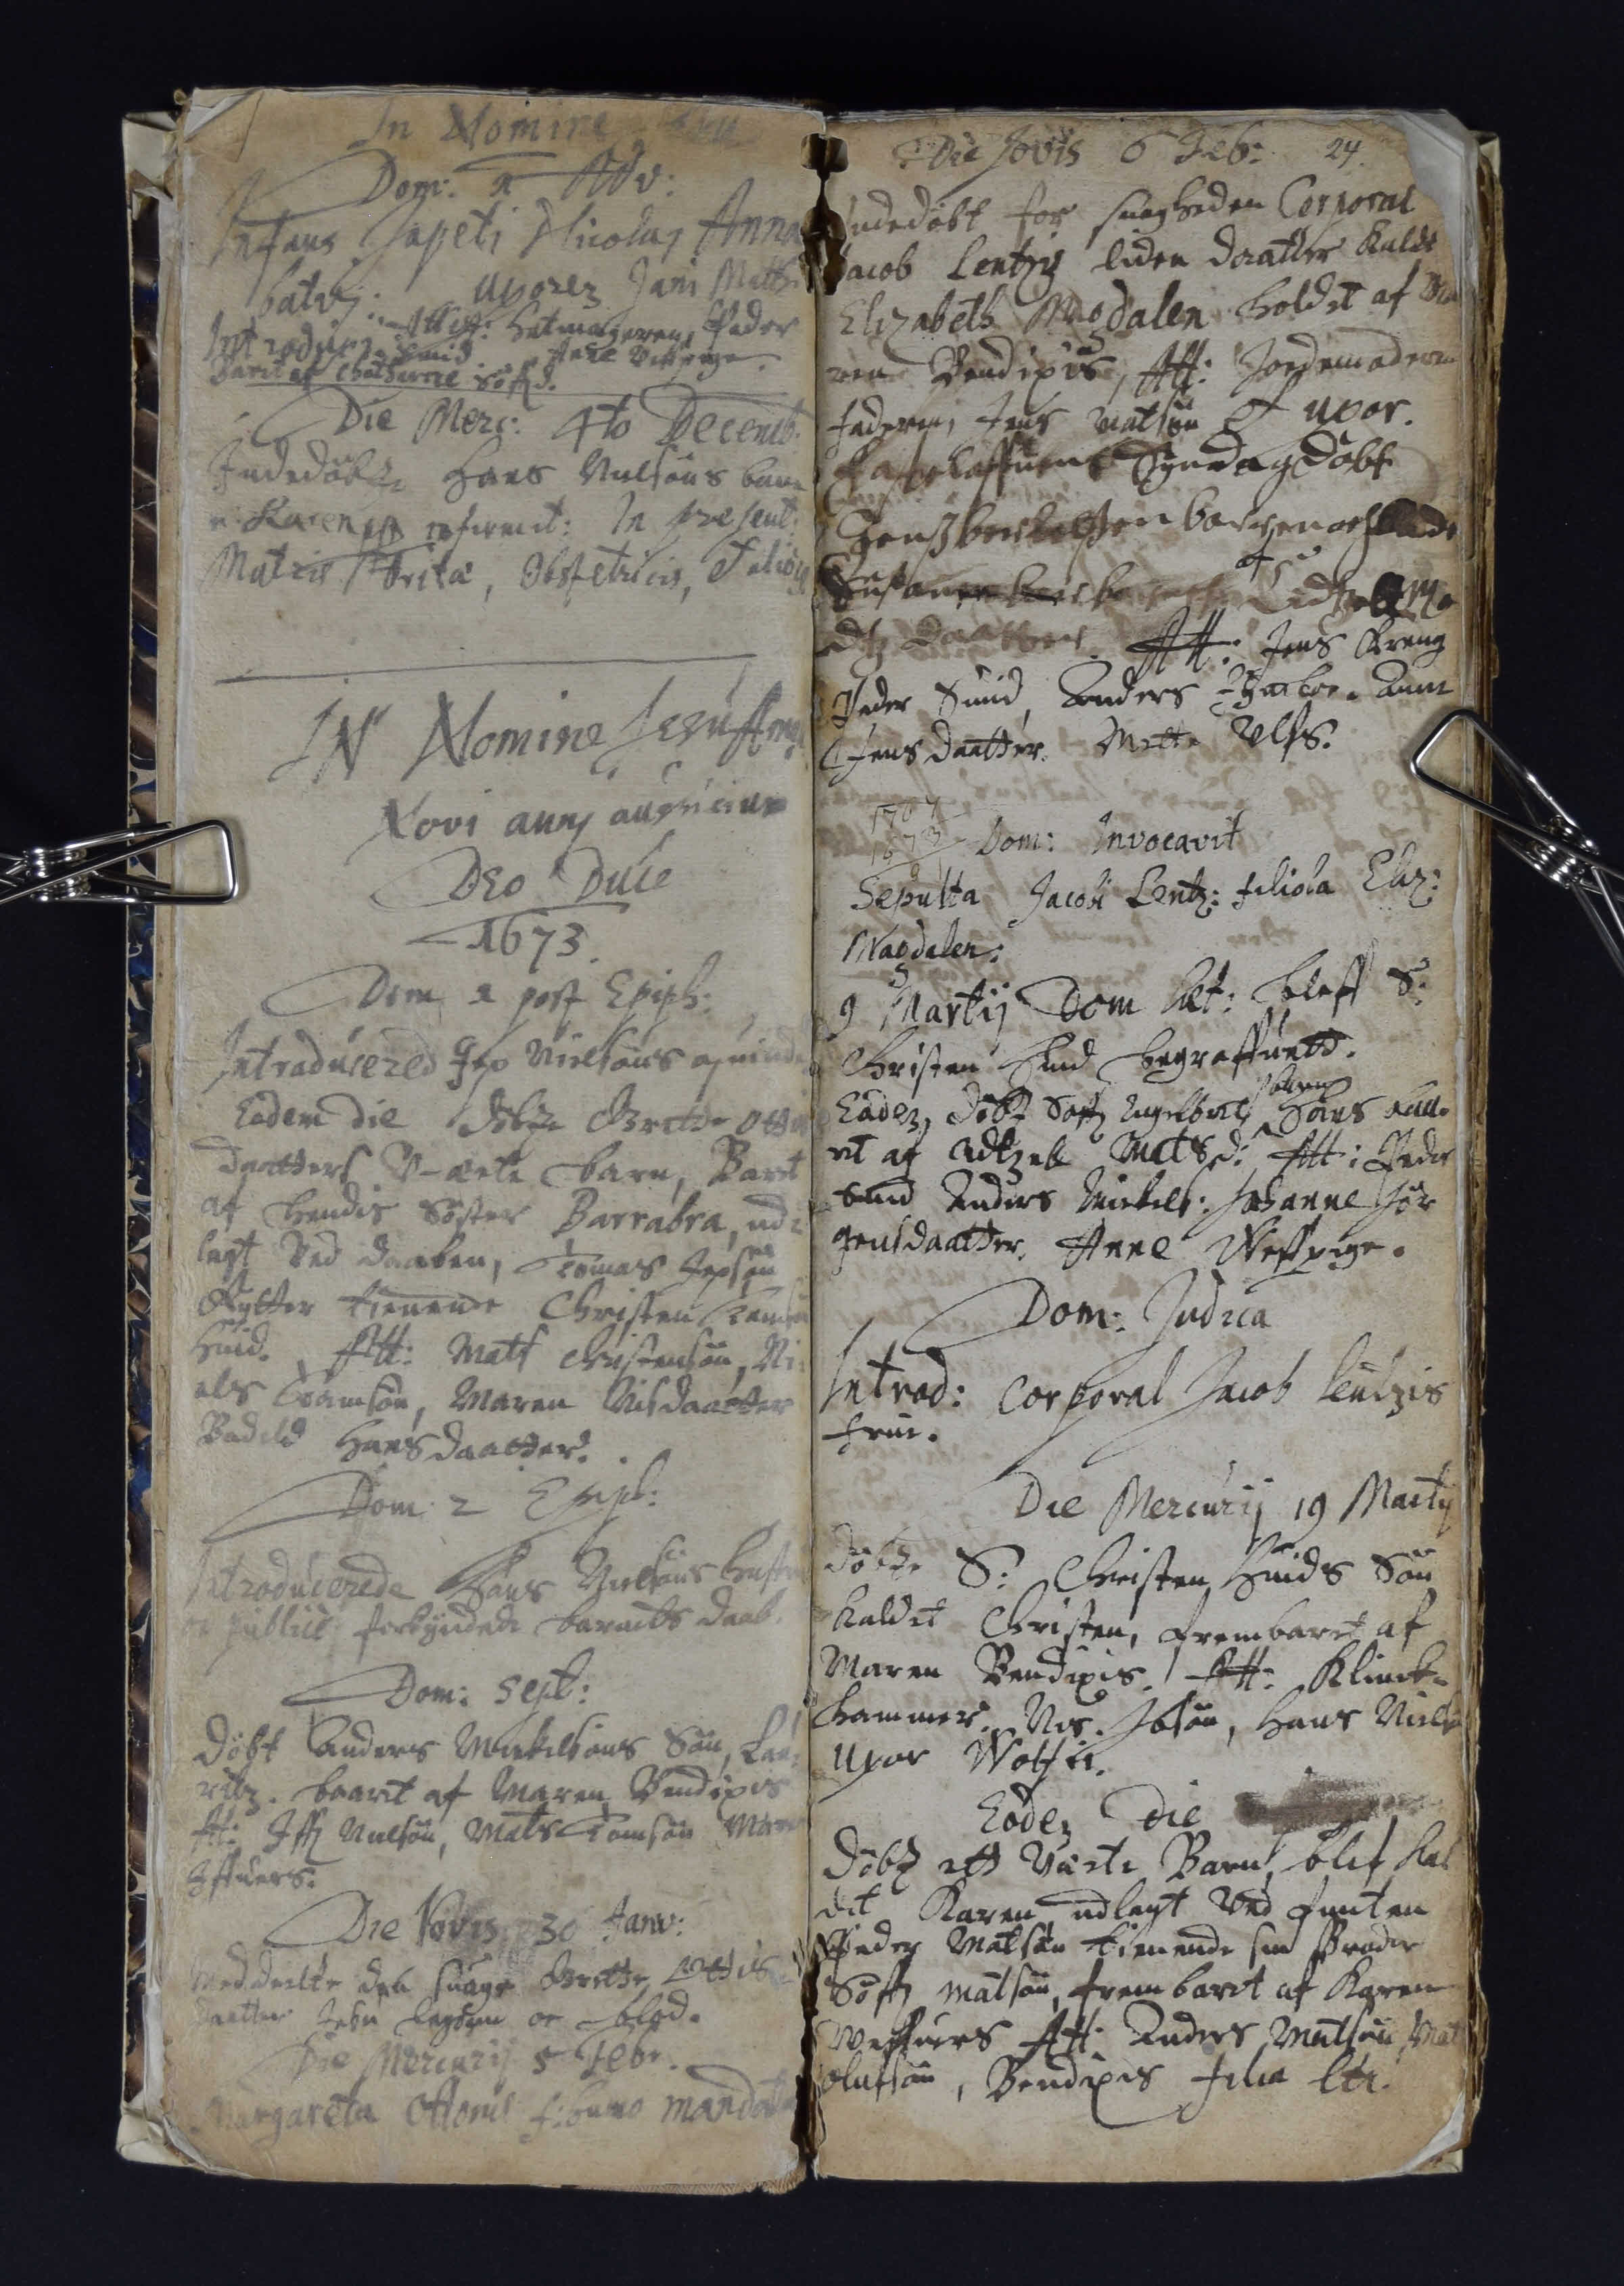In Nomine
Dom. 1 Adv: 
Infans Japeti Nicolaj Anna
uxorez Jani Matthiæ
batis i attest: Hatmaggeren. Peder
Introduci##.
Smid. Jens Ulfs pige
Karen oc Chatherine Søffd.
Die Merc: 4to Decemb
Indedøbte Hans Nielsøns barn
n: Karen, pp infirmit: In present:
Matris Hvita, Obstetricis,
IN Nomine  Jesu
Xovi anni
1673
DEO
Dom. 1 post Epiph:
Introducered Jep Nielsøns qvinde.
Eodem Die døbte Grette Ottis
daatters V-acte barn, Baret
af hindes Søster Barrabra, ud¬
lagt ved daaben, Thomas Jepsøn
Rytter tienende Christen Thamsøn
Hvid. Att: Mats Christensøn, Ni¬
els Tamsøn, Maren Nisdaatter
Bodeld Hansdaatter.
Dom. 2 Epiph:
IntroduceredeHans Nielsøns Hustru
oc publice for##yndeds barnets Daab.
Dom: Sept:
Døbt Anders Mickelsøns Søn, Lau¬
ritz. baaret af Maren Bendixis.
At: Iff Nielsøn, Mats Tomsøn. Maren
Iffuers.
Die Jovis. 30 Janv:
Meddelte den suage Grette Ottis
daatter Jesu Legme oc blod.
Die Mercuris. 5 Febr.
Margareta Ottosd. fibumo mandat 
24.
Die Jovis. 6 Feb:
Indedøbt for suagheden Corporal
Jacob Lentzis liden daatter kaldet
Elizabeth Magdalen holdet af Ma
ren Bendixis. Att: Jordemoderen
Faderen, Jens Matsøn et uxor
Fastelaffuens Syndag Døbt
Jenss Berthelsøns barren och
æt 5
Susanne bar barnet Cidzell Ma
dtz Daatter.
Att: Jens Kreng
Peder Smid. Anders ##arlov. Anna
Jensdaatter. Mette Ulfs.
Dom. Invocavit
Sepulta Jacobi Lentz: filiola Eliz:
Magdalen: 
9 Martij Dom. Alt: Bleff S:
Christen Hvid begraffuett.
s##rup
Eodem døbt Søff Engelbrec Hans baa¬
ret af Zidtzel Matsd. Att: Peder
Smed Anders Mickels: Johanne Jør
gensdaatter. Anne Weffpige.
Dom. Judica. 
Introd: Corporal Jacob Lentzis
Frue.
Die Mercurij 19 Martij
Døbte S: Christen Huids Søn
kaldet Christen, frembaret af
Maren Bendixis. Att: Klinck¬
hammer. Nis. Ibsøn, Hans Nielsøn
uxor Wolfis.
Eodem Die
Døbt ett Uacte Barn blef kal
det Karen, udlagt ved funten
Peder Matsøn tienende sin Broder
Søffr Matsøn, frembaret af Karen
Weffuers. Att: Anders Matsøn. Mat
Olufsøn, Bendixis filia etc.
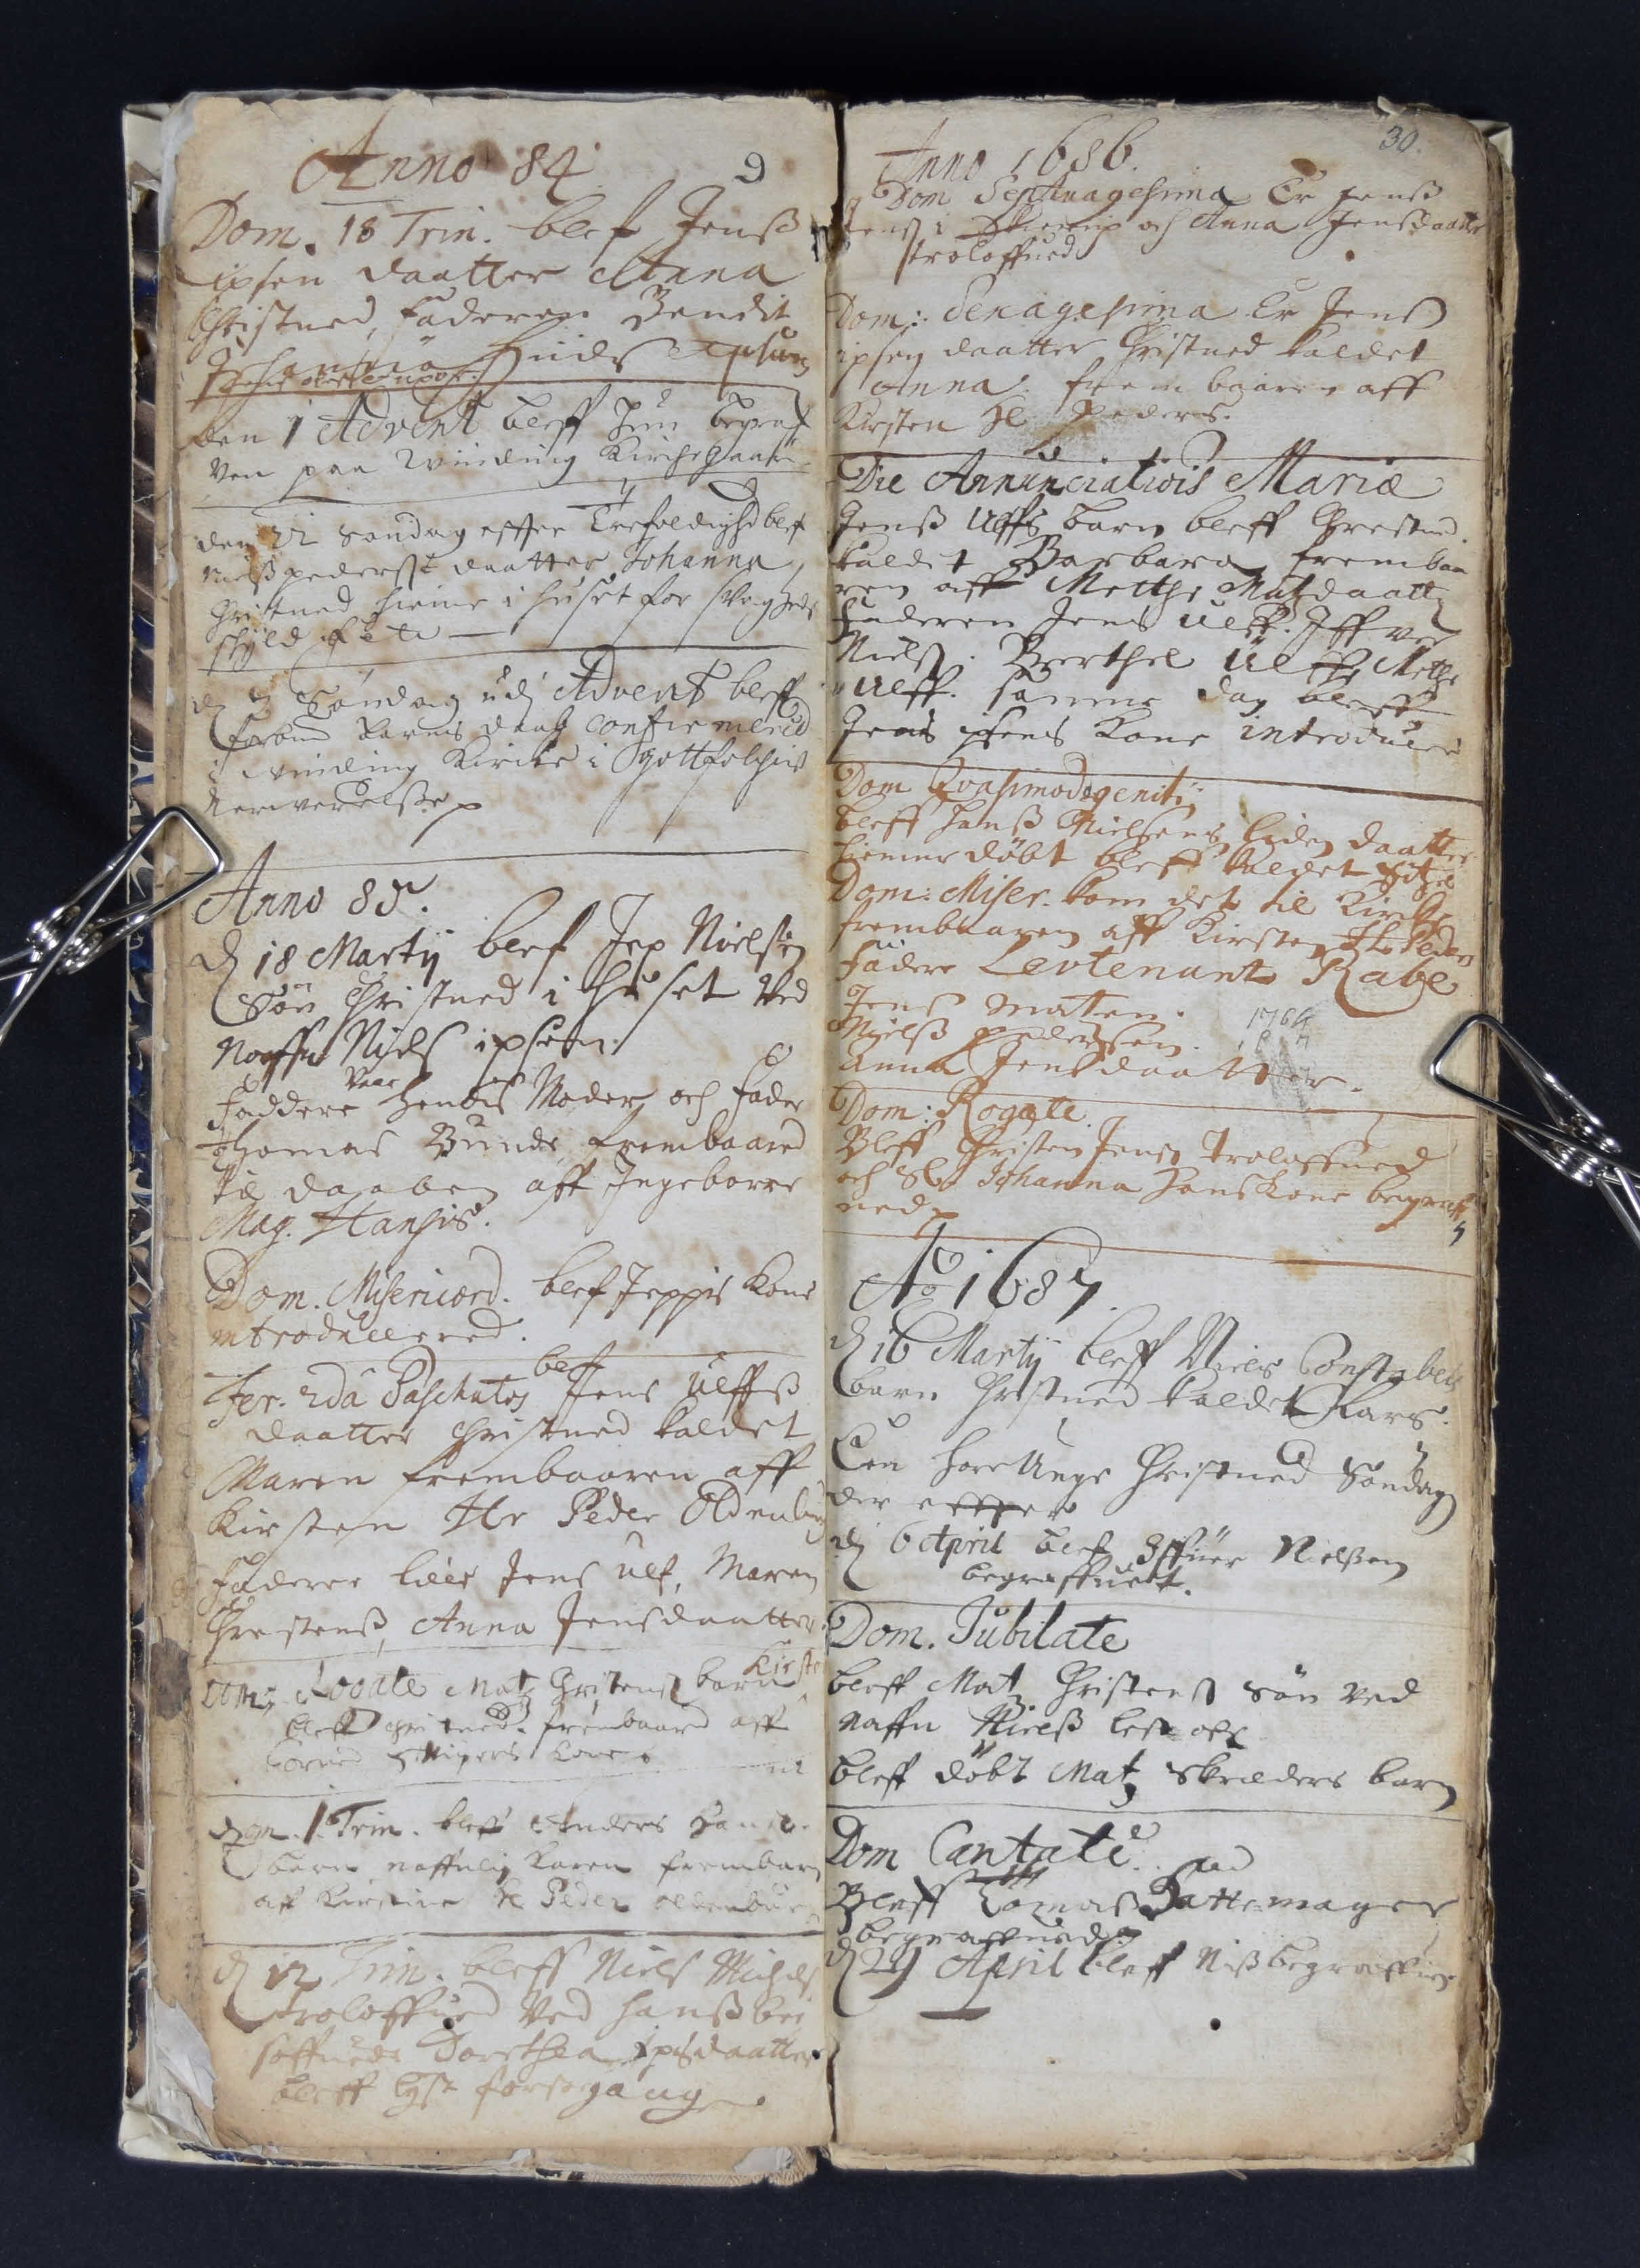Anno 84
Dom. 18 Trin. blef Jenss
Ipsen daatter Anna
Christned, Faderene Bendit
Johanna Huids
## ## ## uxor##
Den 1 Advent bleff Hun begraff
ven paa Winding Kirchegaar
den 22 Sondag efter Trefoldighed. blef
Nielss Peders daatter Johanna
Christned hieme i huuset for svagheds
skyld ## etc
d 2 Søndag udj Advent. bleff
forbem Barns daab confirmered
i Vinding Kirche i gottfolchis
nerverelsse
Anno 85.
d 18 Martij. blef Jep Niels
Søn Christned i huset ved
Naffn Niels ipsen.
Vaar
Faddere hendis Moder och Fader
Thomas Bundes, frembaared
til daaben aff Ingeborre
Mag. Hansis.
Dom. Misericord. blef Jeppis Kone
introducerred
bleff
Fer. 2da Paschatos Jens Ulffss
daatter christned kaldet
Maren frembaaren aff
Kirsten Hr Peder Oldenborg
Faderne lille Jens Ulf, Maren
Christens. Anna Jensdaatter
Kirste
Rogate. Matz Christens barn
bleff christned, frembaaren aff
Skipers Kone
Dom. 1. Trin. bleff Anders Hans
barn naffnlig Karen frembaaren
aff Kirstine Hl Peder Oldebor## 
d 12 Trin bleff Niels Michels
troloffued Ved hanss beei
soffuede Dorthea Ipsdaatter.
bleff lyst forste gang
30
Anno 1686.
Dom Septuagesima Er Jenss
Jens i Skierup och Anna Jensdaatter
troloffued
Dom: Sexagesime. Er Jens
ipsen daatter christned kaldet
Anna frembaaren aff
Kirsten Hl Peders
Die Annunciatiois Mariæ
Jenss Ulffs barn bleff chrestned
kaldet Barbara frembaa
ren af Metthe Matzdaatt
Faderen Jens Ulff. Iffver
Niels. Berthel Ulff. Methe
Ulff. Samme dag bleff
Jens ipsens kone introducered
Dom. Qvasimodogeniti
bleff Hanss Nielsens liden daatter
hiemmedøbt blef kaldet Sitzel
Dom: Miser. kom det til Kirche
frembaaren aff Kirsten Hl Peders
Fadere Levtenant Rabe
Jens Matzen.
Nielss Pedersen.
Anna Jensdaatter
Dom: Rogate
Bleff Christen Jens troloffued
och Sl Johanna Hans Kone begraff¬
ued
Ao 1687
d 16 Martij bleff Niels Constabels
barn christned kaldet Lars.
Een hore Unge christned Søndag
der effter
d 6 April. blef Iffuer Nielsson
begraffuet
Dom. Jubilate
bleff Matz Christens Søn ved
naffn Nielss
bleff døbt Matz Skræders barn
Dom Cantate
Bleff Thomas Hattemager 
begraffued
d 29 April bleff Niss begraffued